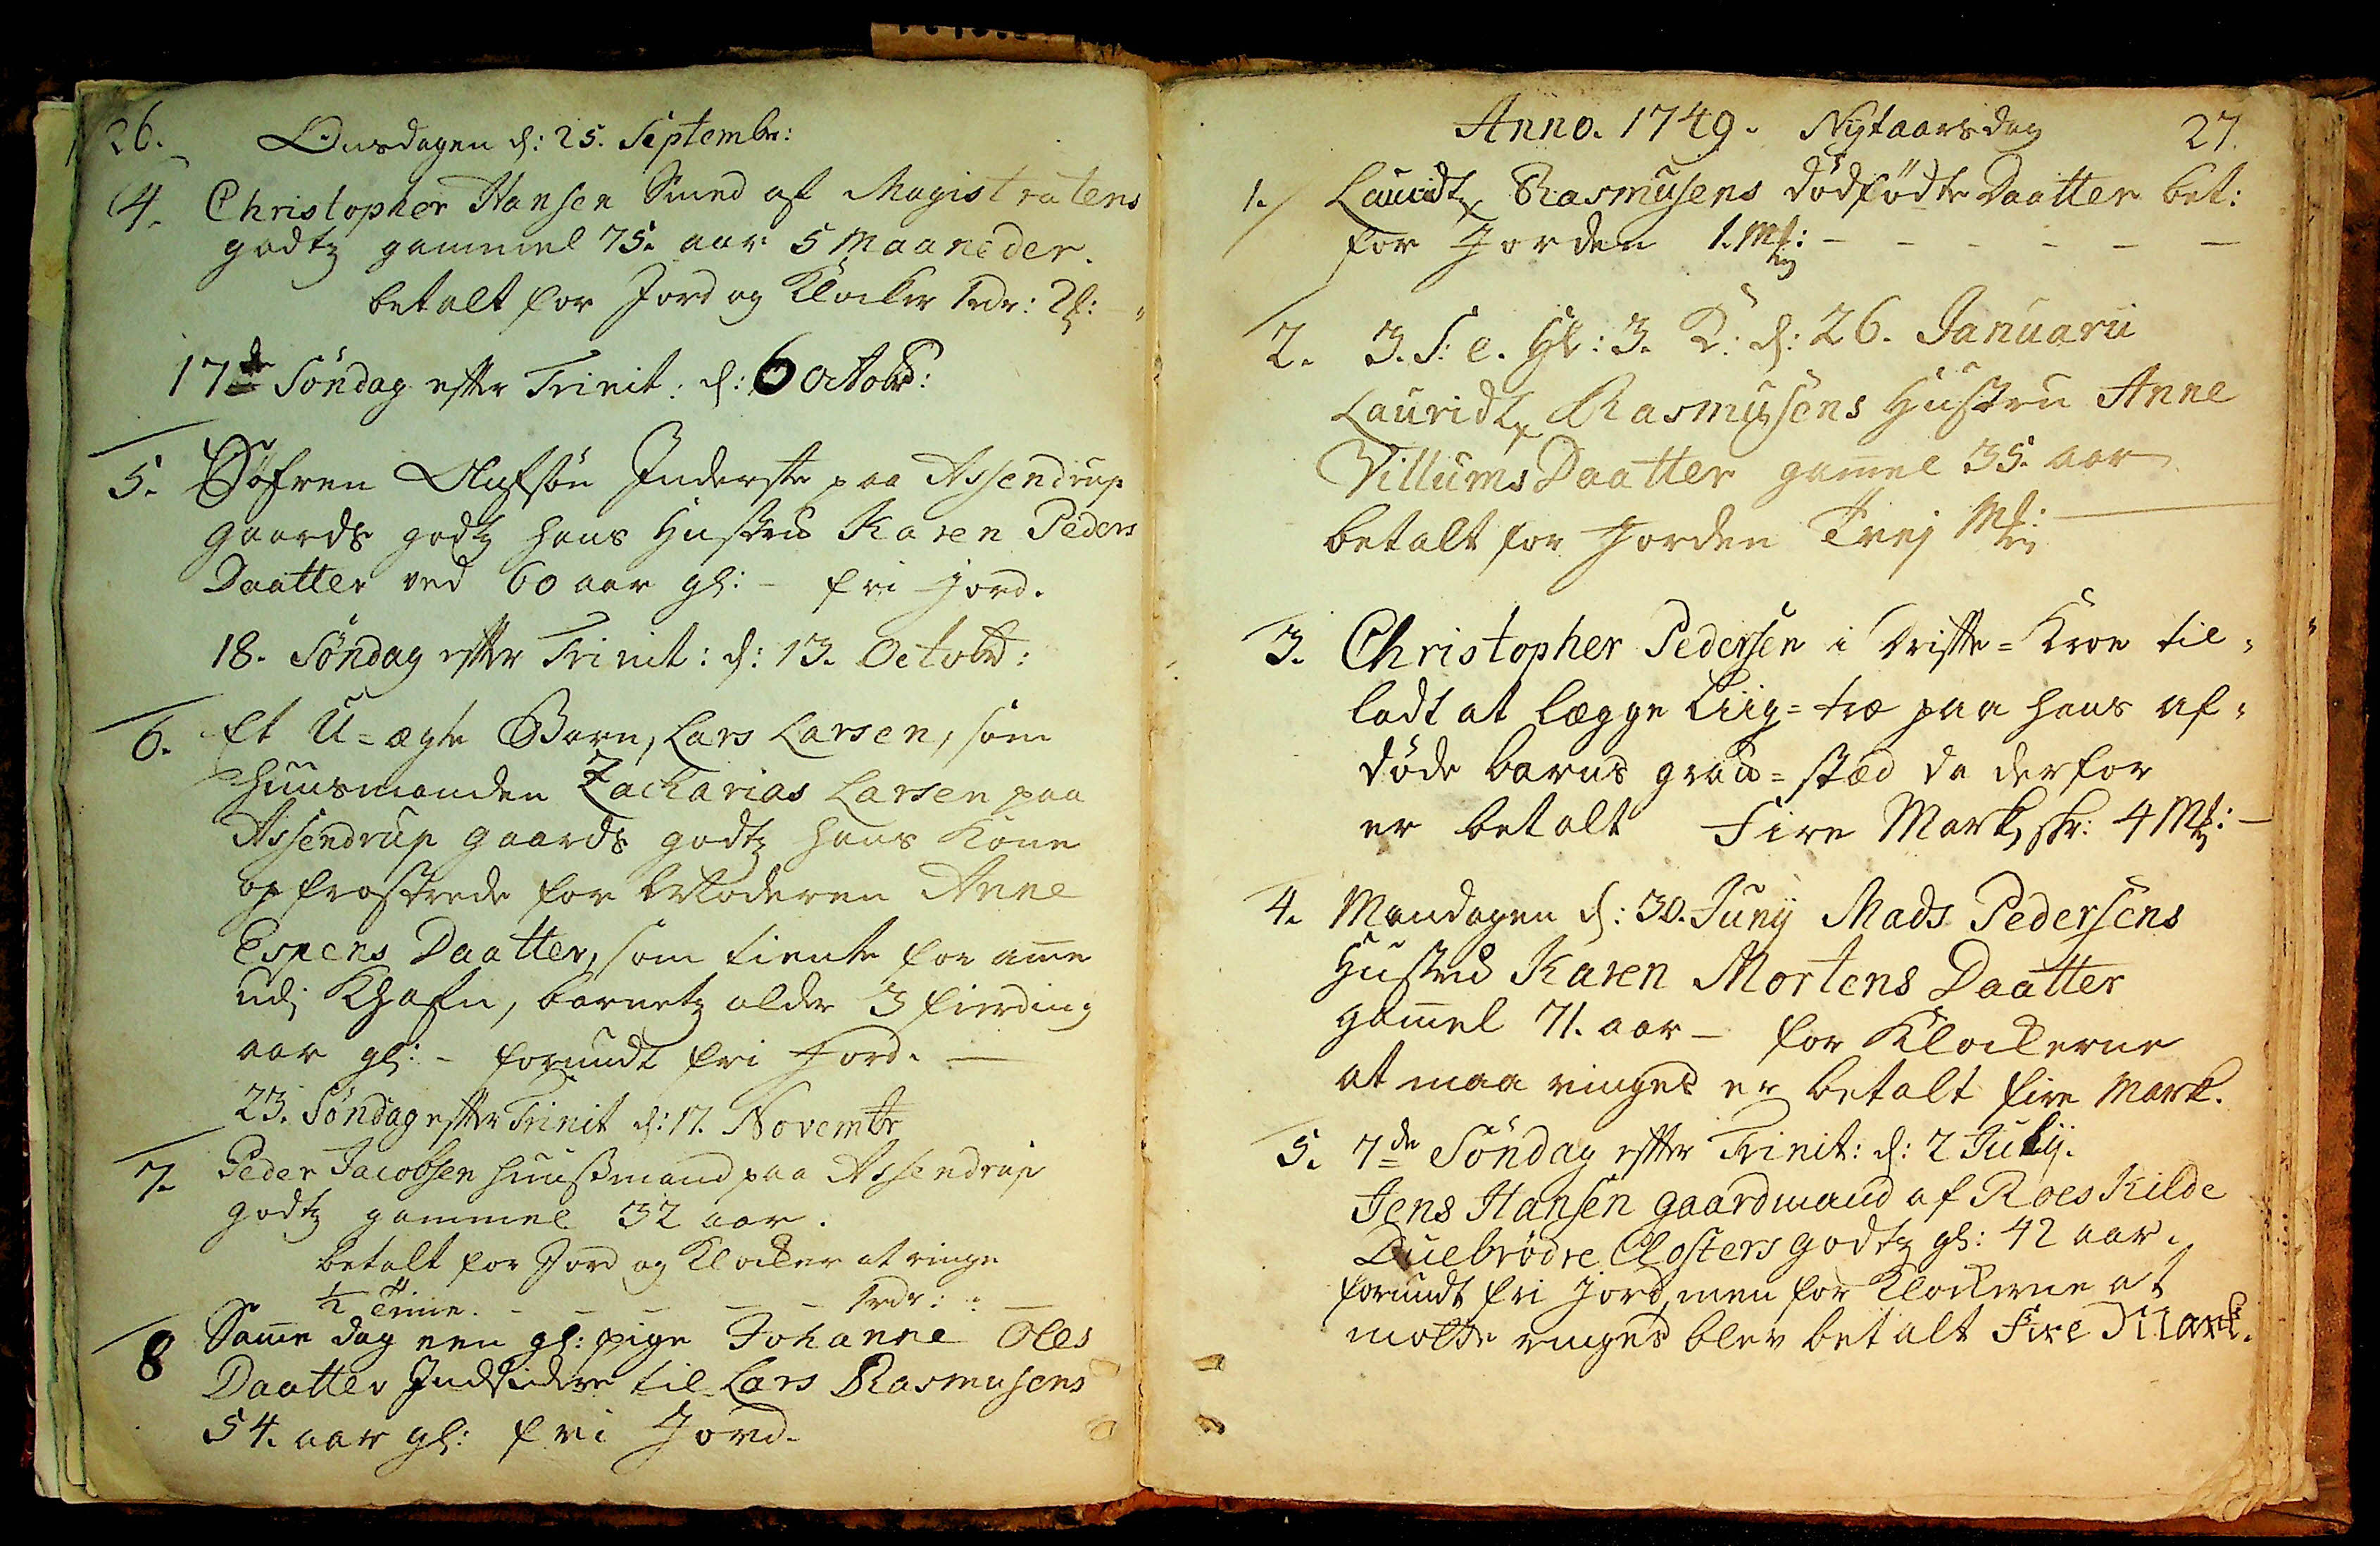26.
Onsdagen d: 20. Septembr:
4. Christopher Hansen Smed af Magistratens
Godtz gammel 75. aar 5 Maaneder.
betalt for Jord og Klocker 1rdr: 2m: - =
17de Søndag efter Trinit: d: 6 Octbr:
5. Søfren Olufsøn Inderste paa Assendrup
Gaards godtz hans Hustru Karen Peders
Daatter ved 60 aar gl: - fri Jord.
18. Søndag efter Trinit: d: 13. Octobr:
6. Et U=ægte Barn, Lars Larsen, som
Huusmanden Zacharias Larsen paa
Assendrup Gaards Godtz hans Kone
opfrostrede for Moderen Anne
Espens Daatter, som tiente for amme
udi Khafn, Barnetz alder 3 fierding
aar gl: - forundt fri Jord. -
23. Søndag efter Trinit d: 17. Novembr
7. Peder Jacobsen huusmand paa Assendrup
godtz gammel 32 aar.
betalt for Jord og Klocker at ringe
½ Time.
1rdr: 
8. Samme dag een gl: pige Johanne Oles
Daatter Indsidere til Lars Rasmusens
54. aar gl: fri Jord.
27.
Anno 1749.Nytaarsdag
1. Lauridtz Rasmusens dødfødte Daatter bet:
for Jorden 1.mk:
2. 3. S: e. Hl: 3. K: d: 26. Januarii
Lauridtz Rasmusens Hustru Anne
Villums Daatter gammel 35. aar
betalt for Jorden Trej Mk:
3. Christopher Pedersen i Drifte=Kroe til¬
ladt at lægge Liig=træ paa hans af¬
døde barns grau=stæd da derfor
er betaltFire Mark, skr: 4 Mk: -
4. Mandagen d: 30. Junij Mads Pedersens
Hustru Karen Mortens Daatter
gammel 71. aar - for Klockerne
at maa ringes er betalt fire Mark.
5. 7de Søndag efter Trinit: d: 2 Julij.
Jens Hansen Gaardmand af RoesKilde
Duebrødre Closters Godtz gl: 42 aar.
forundt fri Jord, men for Klockerne at
motte ringes blev betalt Fire Mark.
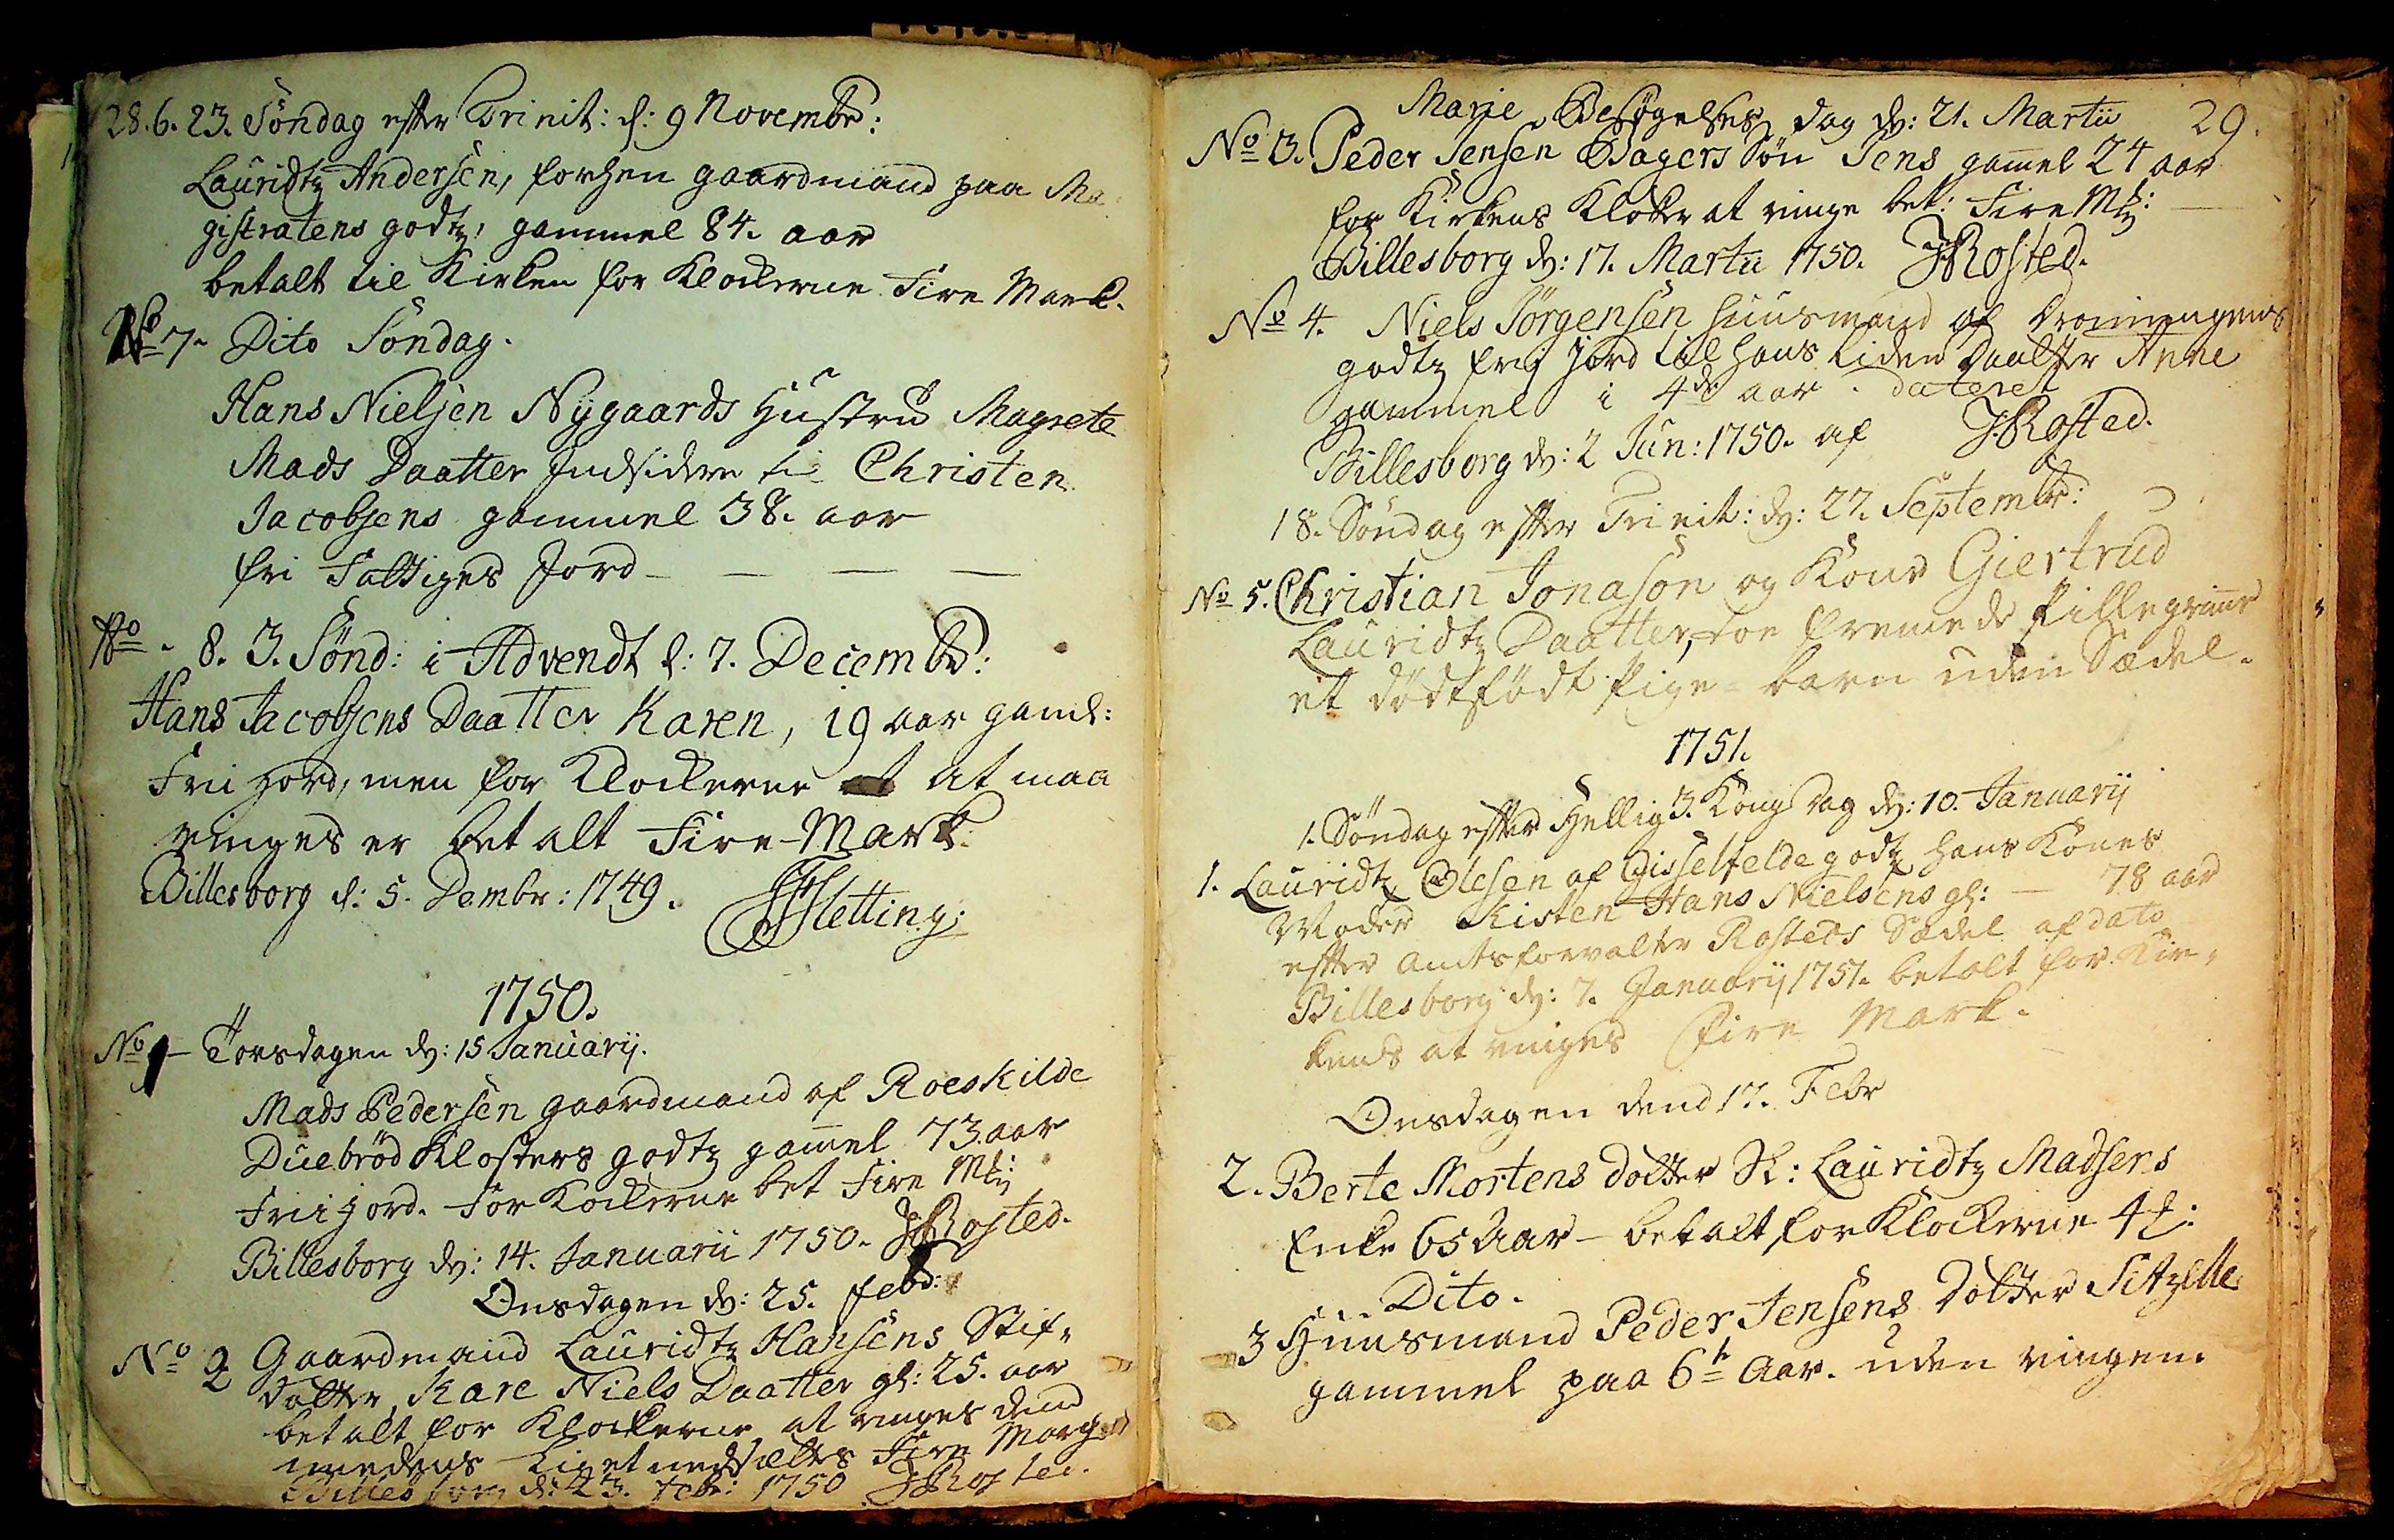28.
6. 23. Søndag efter Trinit: d: 9 Novembr:
Lauridtz Andersen, forhen Gaardmand paa Ma
gistratens Godtz, gammel 84. aar
betalt til kirken for Klockerne. fire Mark.
No 7. Dito Søndag.
Hans Nielsen Nygaards Hustru Magrete
Mads Daatter Jndsidere til Christen
Jacobsens gammel 38. aar
fri Fattiges Jord
No 8. 3. Sønd: i Advendt d: 7. Decembr:
Hans Jacobsens Daatter Karen, 19 aar gaml:
Fri Jord, men for Klockerne at at maa
ringes er betalt Fire-Mark.
Billesborg d: 5. Dcmbr: 1749. JFHetting.
1750.
No 1 Torsdagen d: 15 Januarij.
Mads Pedersen Gaardmand af Roeskilde
Duebrød Klosters Godtz gammel 73. aar
Frijord. For Kockerne bet Fire Mk:
Billesborg d: 14. Januarii 1750. JRosted.
Onsdagen d: 25. febr:
No 2 Gaardmand Lauridtz Hansens Stif¬
datter, Kare Niels Daatter gl: 25. aar
betalt for Klockerne at ringes dend
imedens Liget nedsættes Fire March
Billesborg, d: 23. febr: 1750 JRosted.
29.
Marie Besøgelses dag d: 21. Martii
No 3. Peder Jensen Bagers Søn Jens gammel 24 aar
for Kirkens Klocke at ringe bet: Fire Mk:
Billesborg d: 17. Martii 1750. JRosted.
No 4. Niels Jørgensen Huusmand af Dronningens
Godtz fri Jord til hans liden Daatter Anne
gammel i 4de aar. dateret
Billesborg d: 2 Jun: 1750. af J.Rosted.
18. Søndag efter Trinit: d: 27. Septembr:
No 5. Christian Jonasøn og Kone Giertrud
Lauridtz Daatter, toe fremede Pillegrimme
et dødtfødt Pige=barn uden Sædel.
1751.
1. Søndag efter Hellig 3. Kong dag d: 10. Januarij
1. Lauridtz Olesen af Gisselfelde godtz hans Kones
Moder Kirsten Hans Nielsens gl: - 78 aar
efter Amtsforvalter Rosteds Sædel af dato
Billesborg d: 7. Januarij 1751. betalt for kir=
kens at ringes Fire Mark.
Onsdagen dend 17. Febr
2. Berte Mortens dotter Sl: Lauridtz Madsens
Enke 65 Aar - betalt for klockerne 4m.
Dito.
3. Huusmand Peder Jensens Dotter Sitzelle
gammel paa 6te Aar. uden ringen